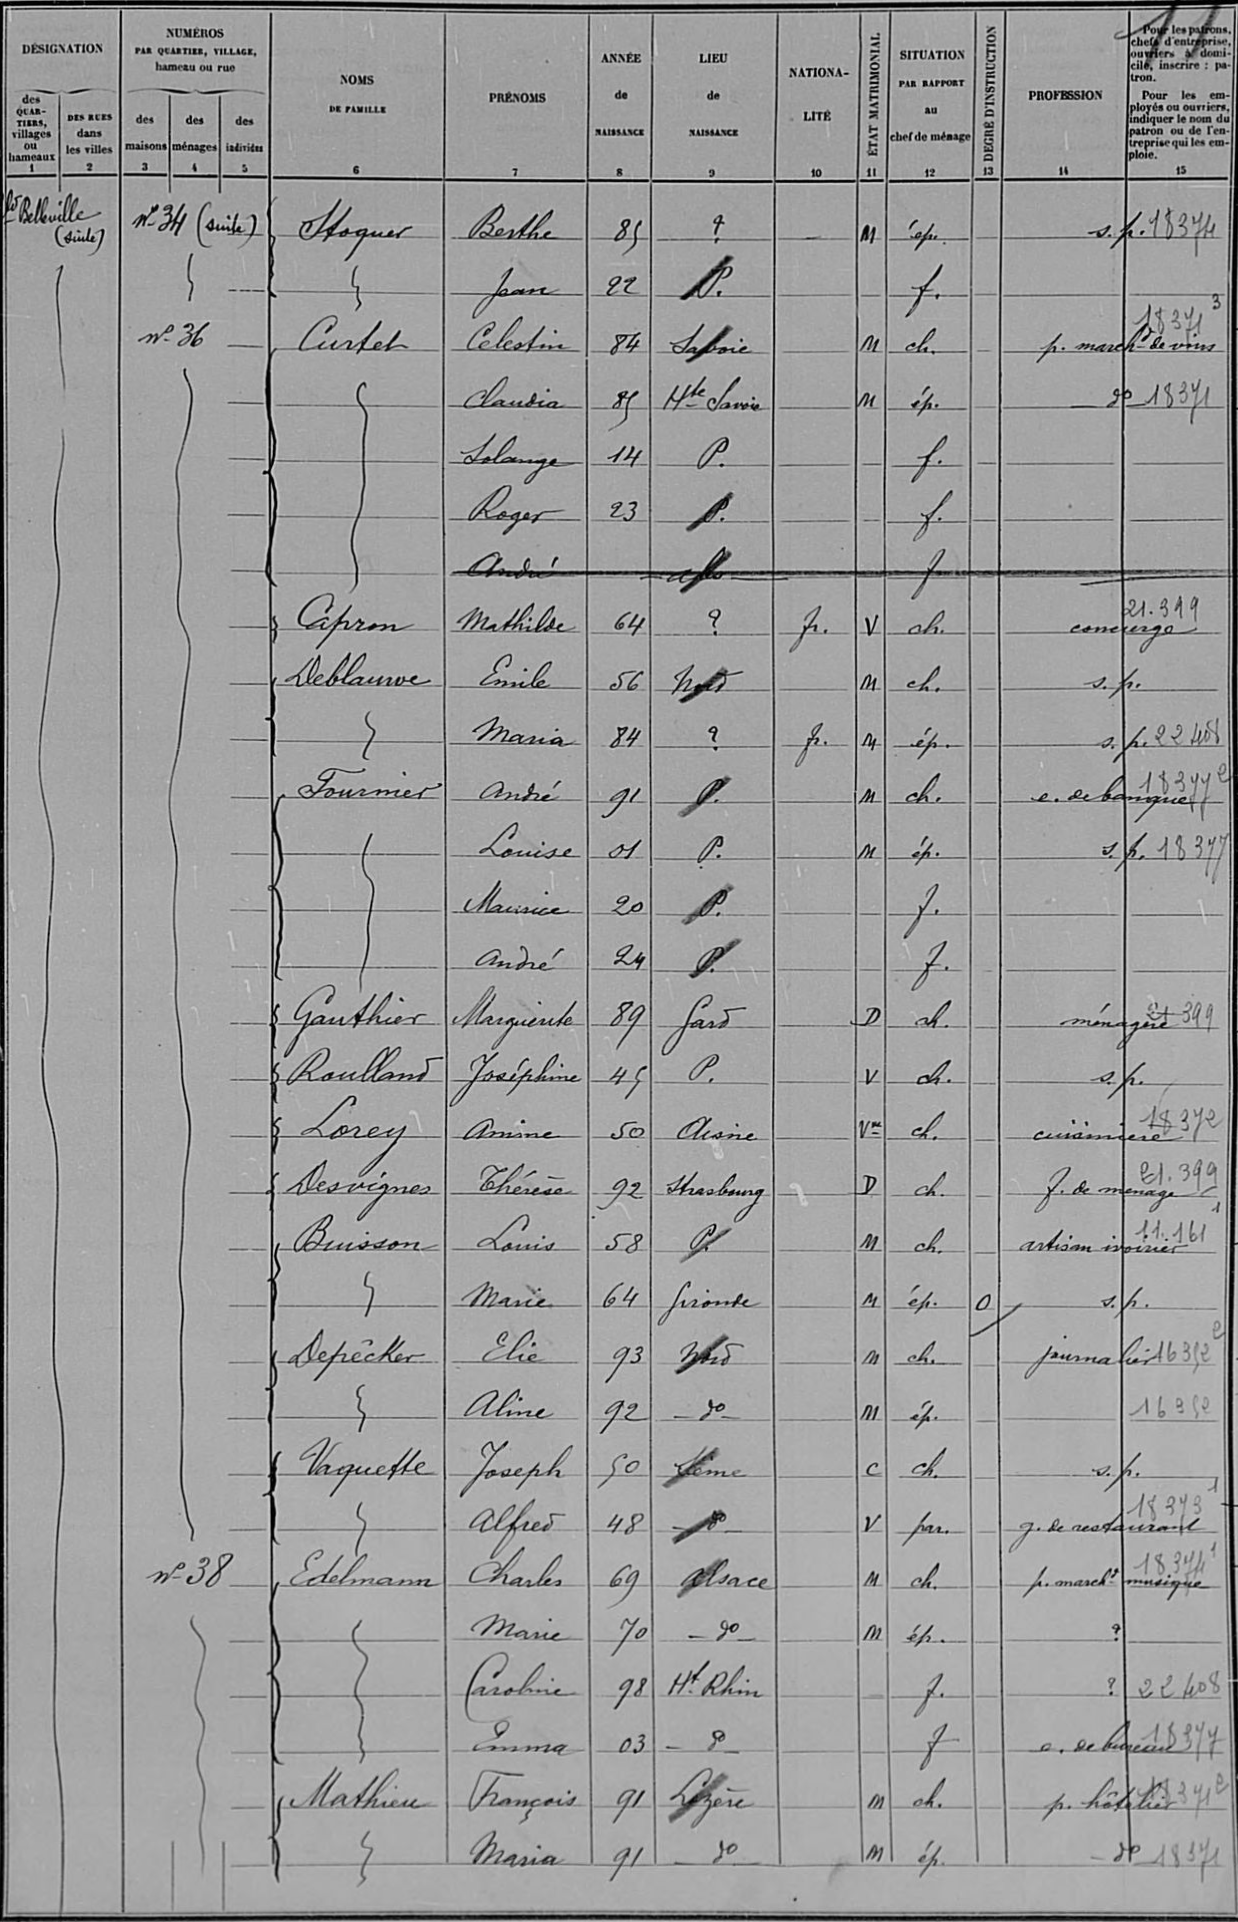Hoquer/Berthe/85/¤/¤/M/ép /¤/s pr /18374
¤/Jean/22/P /¤/¤/f /¤/¤/¤/¤
Curhet/Celestine/84/Savoie/¤/M/ch /¤/p marchd de vins/¤
¤/Claudia/85/Hte Savoie/¤/M/ép /¤/d°/18371
¤/Solange/14/P /¤/¤/f /¤/¤/¤/¤
¤/Roger/23/P /¤/¤/f /¤/¤/¤/¤
¤/André/¤/abs/¤/¤/f /¤/¤/¤/¤
Capron/Mathilde/64/¤/fr /V/ch /¤/concierge/21 399
Deblaurve/Emile/56/Nord/¤/M/ch /¤/s p /¤/¤
¤/Maria/84/¤/fr /M/ép /¤/s pr /22408
Fourmier/André/91/P /¤/M/ch /¤/e de banque/¤
¤/Louise/01/P /¤/M/ép /¤/s p /18377
¤/Maurice/20/P /¤/¤/f /¤/¤/¤
¤/André/24/P /¤/¤/f /¤/¤/¤
Gauthier/Marguerite/89/Gard/¤/D/ch /¤/ménagère/¤
Roulland/Joséphine/45/P /¤/V/ch /¤/s p /¤/¤
Lorey/Amine/50/Aisne/¤/Vve/ch /¤/cuisinière/¤
Desvignes/Thérèse/92/Strasbourg/¤/D/ch /¤/f de ménage/¤
Buisson/Louis/58/P /¤/M/ch /¤/artisan ivoirier/11161
¤/Marie/64/Gironde/¤/M/ép /O/s p /¤/¤
Depêcher/Elie/93/Nord/¤/M/ch /¤/journalier/163522
¤/Aline/92/d°/¤/M/ép /¤/¤/16352
Vaquesse/Joseph/50/Seine/¤/C/ch /¤/s p /¤/¤
¤/Alfred/48/d°/¤/V/par/¤/g de restaurant/¤
Edelmann/Charles/69/Alsace/¤/M/ch /¤/p marchd musique/¤
¤/Marie/70/d°/¤/M/ép /¤/¤/¤/¤
¤/Caroline/98/Ht Rhin/¤/¤/f /¤/¤/22408
¤/Emma/03/d°/¤/¤/f/¤/e de bureau/18377
Mathieu/François/91/Lozère/¤/M/ch /p hôtelier/¤
¤/Maria/91/d°/¤/M/ép /¤/d°/18371
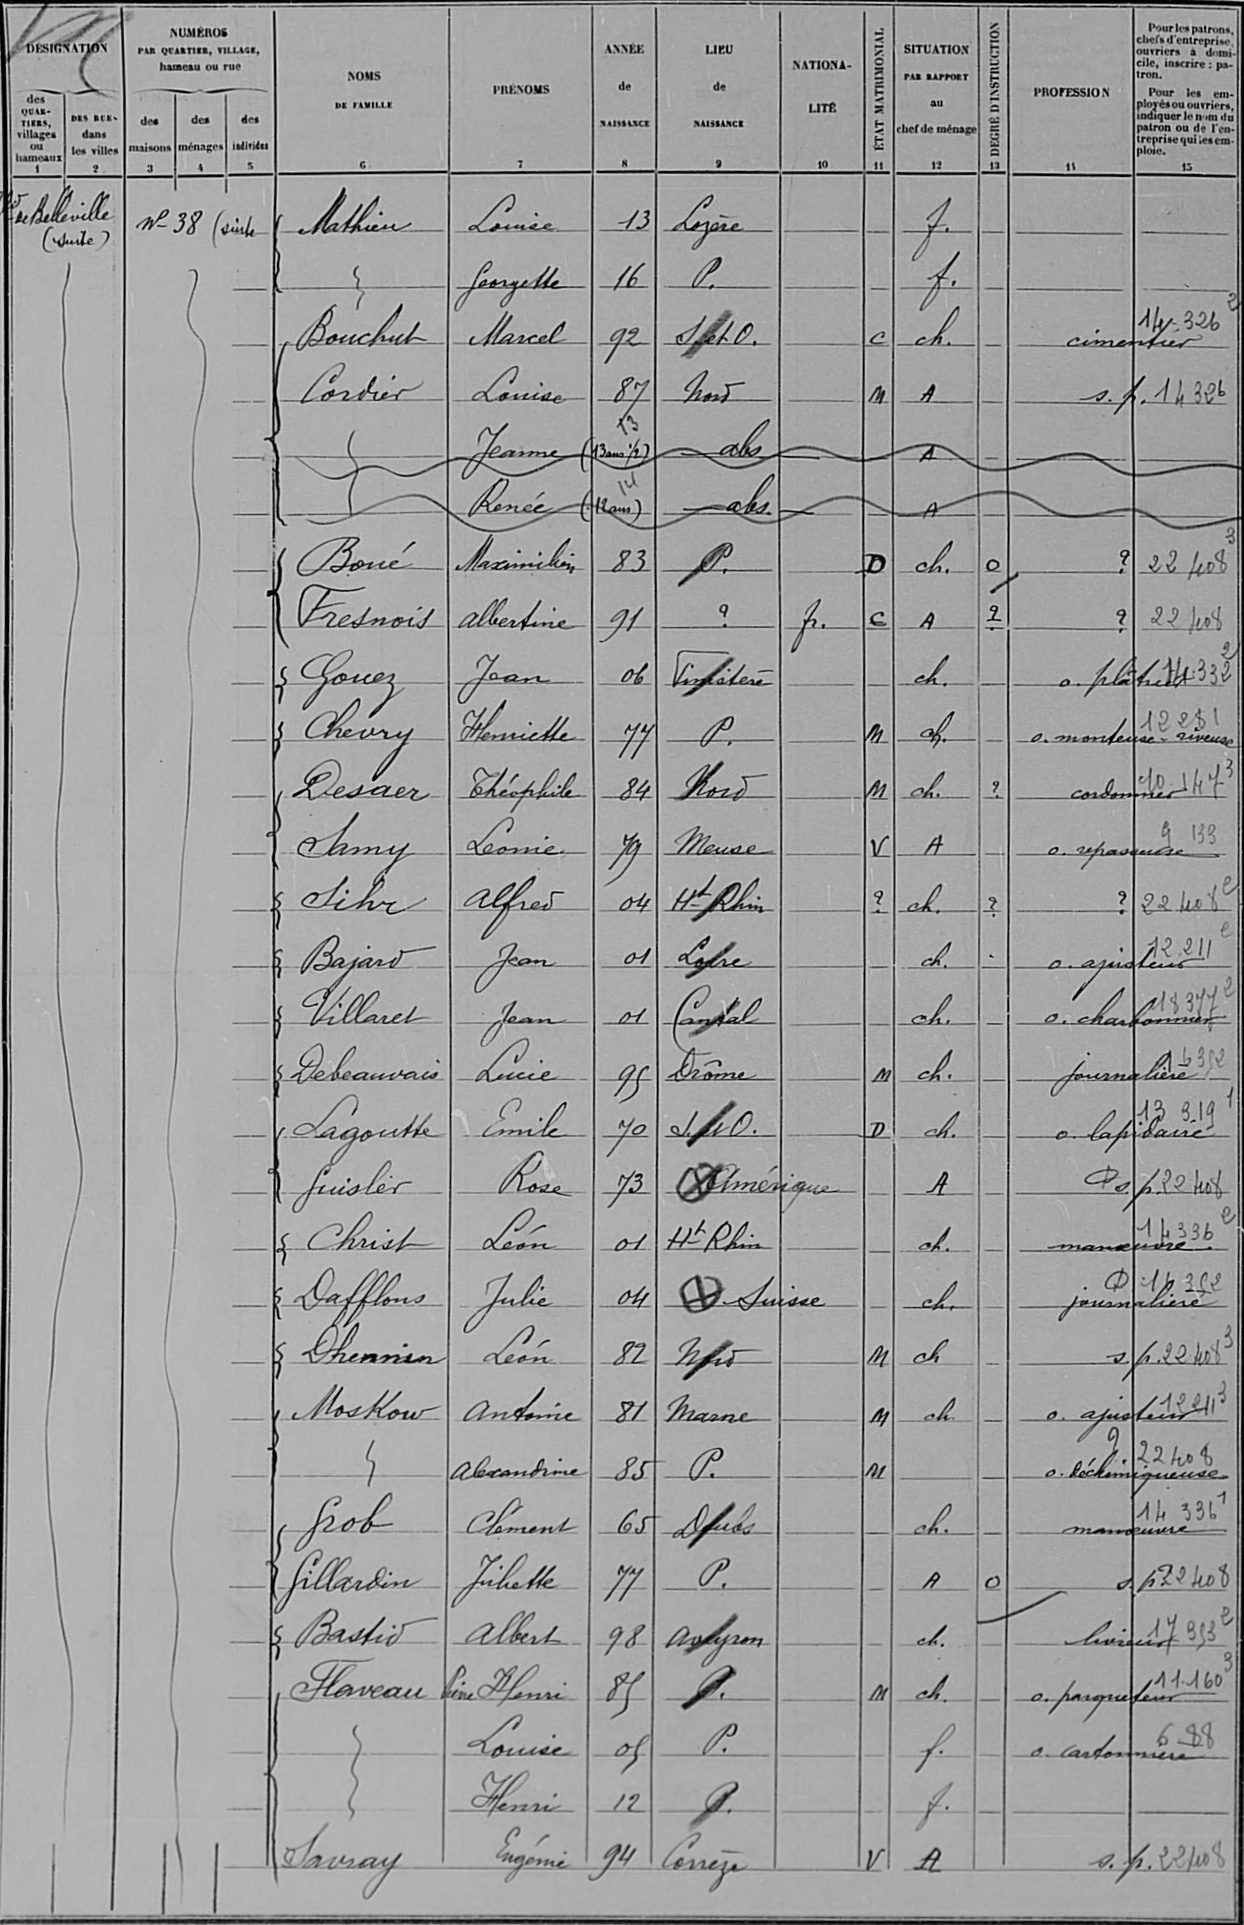Mathieu/Louise/13/lozère/¤/¤/f /¤/¤/¤/¤
¤/Georgette/16/P /¤/¤/f /¤/¤/¤/¤
Bouchut/Marcel/92/S et O /¤/c/ch /¤/cimentier/¤
Cordier/Louise/87/Nord/¤/M/A/¤/s p /14326
¤/Jeanne/(13 ans 1 2) 13/abs/¤/¤/A/¤/¤/¤/¤
¤/Renée/(12 ans) 14/abs /¤/¤/A/¤/¤/3/¤
Boué/Maximilien/83/P /¤/D/ch /O/¤/224083
Fresnois/Albertine/91/¤/fr /C/A/¤/¤/22408
Gouez/Jean/06/Finistère/¤/¤/ch /¤/o plâtrier/143322
Chevry/Henriette/77/P /¤/M/ch /¤/o monteuse riveuse/¤
Desaer/Théophile/84/Nord/¤/M/ch /¤/cordonnier/101473
Samy/Leonie/79/Meuse/¤/V/A/¤/o repaseuse/¤
Sihr/Alfred/04/Ht Rhin/¤/¤/ch /¤/¤/224082
Bajard/Jean/01/Loire/¤/¤/ch /¤/o ajusteur/122112
Villaret/Jean/01/Cantal/¤/¤/ch /¤/o charbonnier/¤
Debeauvais/Lucie/95/Drôme/¤/M/ch /¤/journaliere/16352
Lagousse/Emile/70/S et O /¤/D/ch /¤/o lapidairé/133191
Guisler/Rose/73/Amérique/¤/¤/A/¤/s p /224082
Christ/Léon/01/Ht Rhin/¤/¤/ch /¤/manoeuvre/¤
Dafflons/Julie/04/Suisse/¤/¤/ch /¤/journalière/¤
Dhennin/Léon/82/Nord/¤/M/ch/¤/s p /224083
Moskow/Antoine/81/Marne/¤/M/ch /¤/o ajusteur/122113
¤/Alexandrine/85/P /¤/M/¤/¤/o déchimiqueuse/¤
Grob/Clément/65/Doubs/¤/¤/ch /¤/manoeuvre/¤
Gillardin/Juliette/77/P /¤/¤/A/O/s p /22408
Bashid/Albert/98/Aveyron/¤/¤/ch /¤/livreur/173532
Flaveau/Pierre Henri/85/P /¤/M/ch /¤/o parqueteur/¤
¤/Louise/05/P /¤/¤/f /¤/o cartonnière/¤
¤/Henri/12/P /¤/¤/f /¤/¤/¤/¤
Savray/Eugénie/Corrèze/¤/V/A/¤/s p /22408
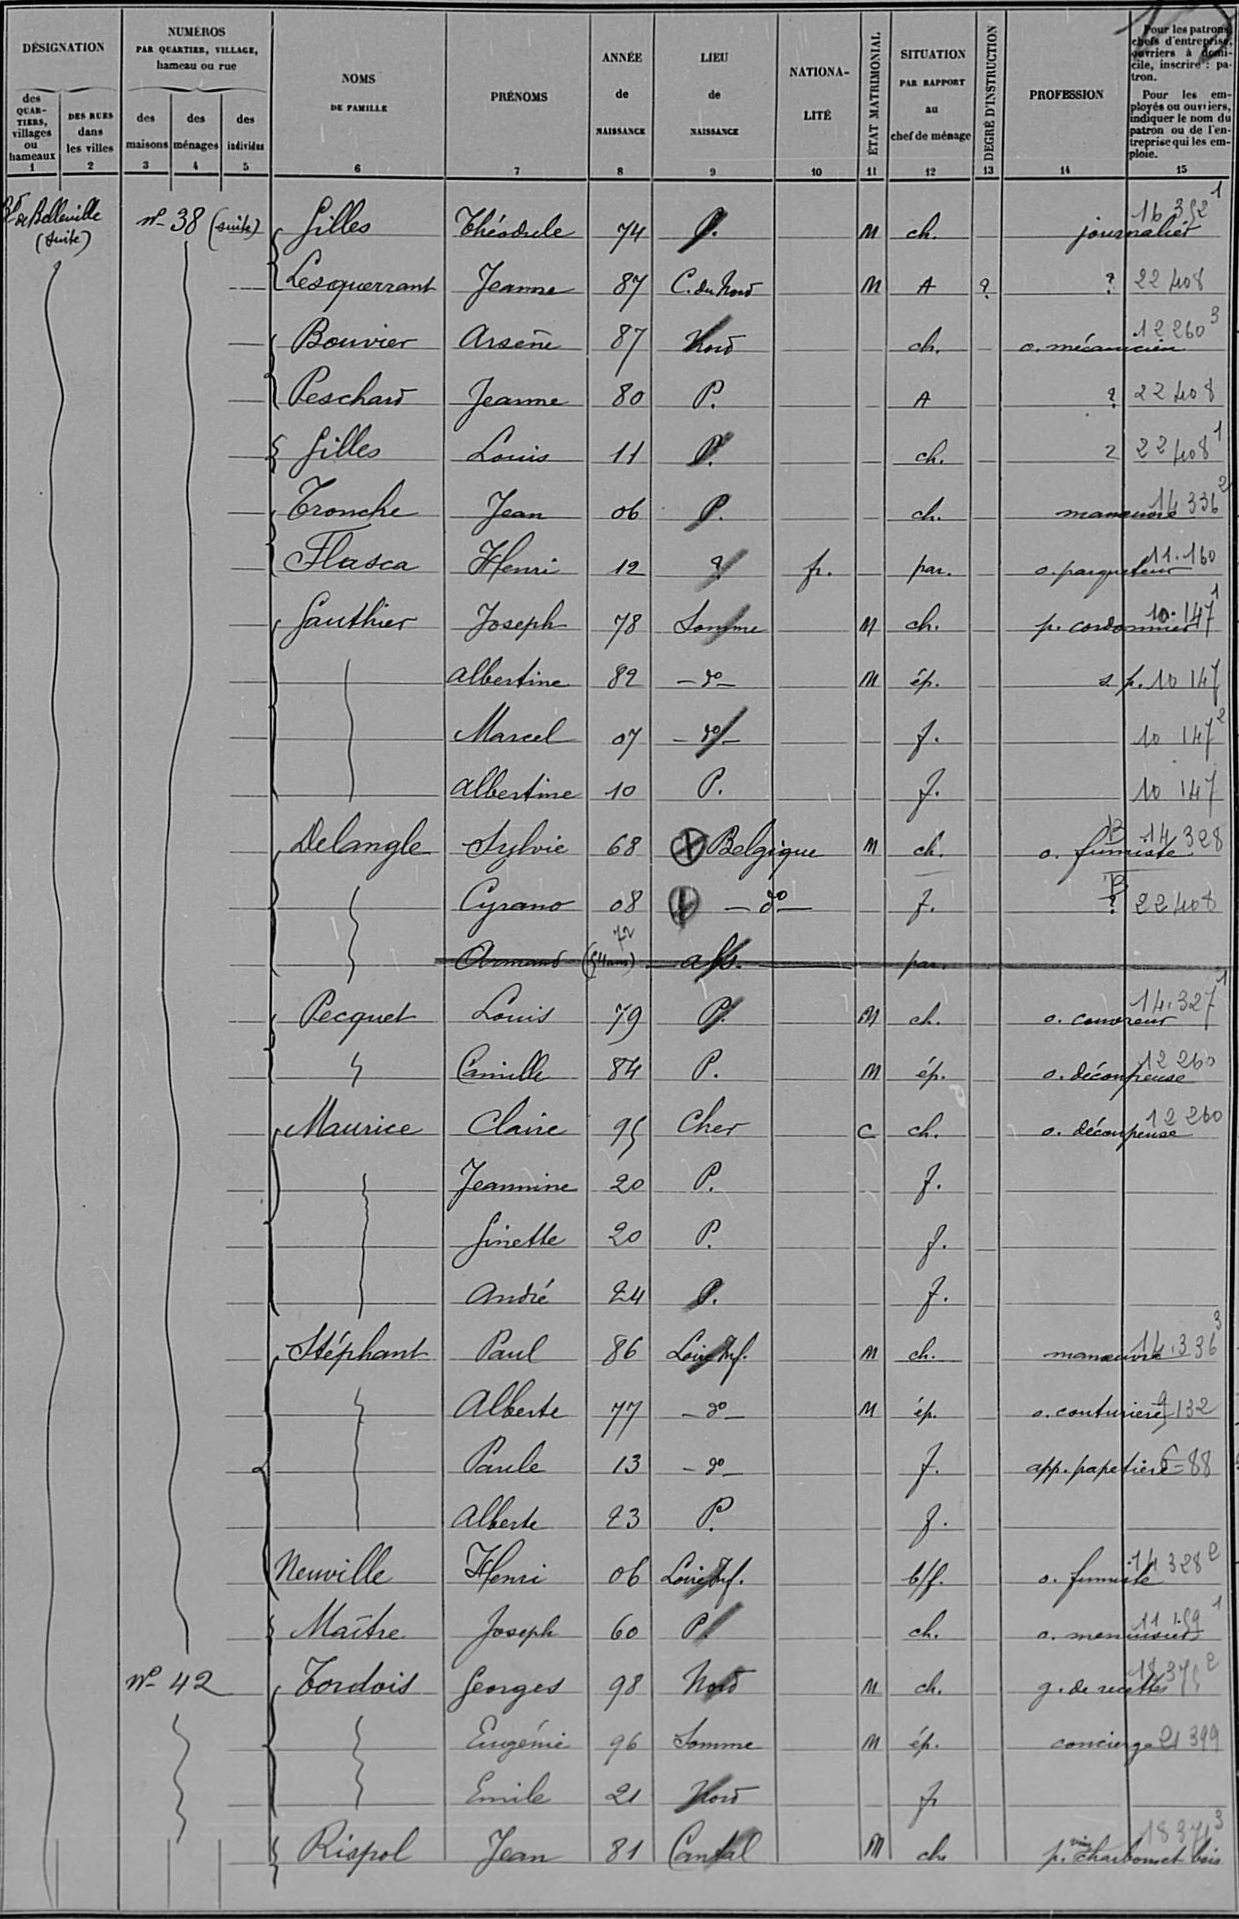Gilles/Théodule/74/P /¤/M/ch /¤/journalié/¤
Lesquerrant/Jeanne/87/C du Nord/¤/M/A/¤/¤/22408
Bouvier/Arsène/87/Nord/¤/¤/ch /¤/o mécanicien/122603
Peschard/Jeanne/80/P /¤/¤/A/¤/¤/22408
Gilles/Louis/11/P /¤/¤/ch /¤/2/224081
Cronche/Jean/06/P /¤/¤/ch /¤/manoeuvre/143362
Flasca/Henri/12/¤/fr /¤/par /¤/o parqueteur/¤
Gauthier/Joseph/78/Somme/¤/M/ch /¤/p cordonnier/¤
¤/Albertine/82/d°/¤/M/ép /¤/s p /10147
¤/Marcel/07/d°/¤/¤/f /¤/¤/101472
¤/Albertine/10/P /¤/¤/f /¤/¤/10147
Delangle/Sylvie/68/Belgique/¤/M/ch /¤/o fumiste/¤
¤/Cyrano/08/d°/¤/¤/f /¤/¤/22408
¤/Armand/(54 ans) 72/abs /¤/¤/par /¤/¤/1
Pecquet/Louis/79/P /¤/M/ch /¤/o couvreur/¤
¤/Camille/84/P /¤/M/ép /¤/o découpeuse/¤
Maurice/Claire/95/Cher/¤/c/ch /¤/o découpeuse/¤
¤/Jeannine/20/P /¤/¤/f /¤/¤/¤/¤
¤/Ginette/20/P /¤/¤/f /¤/¤/¤/¤
¤/André/24/P /¤/¤/f /¤/¤/3
Stéphant/Paul/86/Loire Inf /¤/M/ch /¤/manoeuvre/14336
¤/Alberte/77/d°/¤/M/ép /¤/o couturiere/9132
¤/Paule/13/d°/¤/¤/f /¤/app papetiere/688
¤/Alberte/23/P /¤/¤/f /¤/¤/¤/¤
Neuville/Henri/06/Loire Inf /¤/¤/b-f/¤/o fumiste/¤
Maitre/Joseph/60/P /¤/¤/ch /¤/o menuisier/111591
Tordois/Georges/98/Nord/¤/M/ch /¤/g de recettes/¤
¤/Eugénie/96/Somme/¤/M/ép /¤/concierge/21399
¤/Emile/21/Nord/¤/¤/f/¤/¤/¤/¤
Rispol/Jean/81/Cantal/¤/M/ch /¤/p charbon et bois/¤